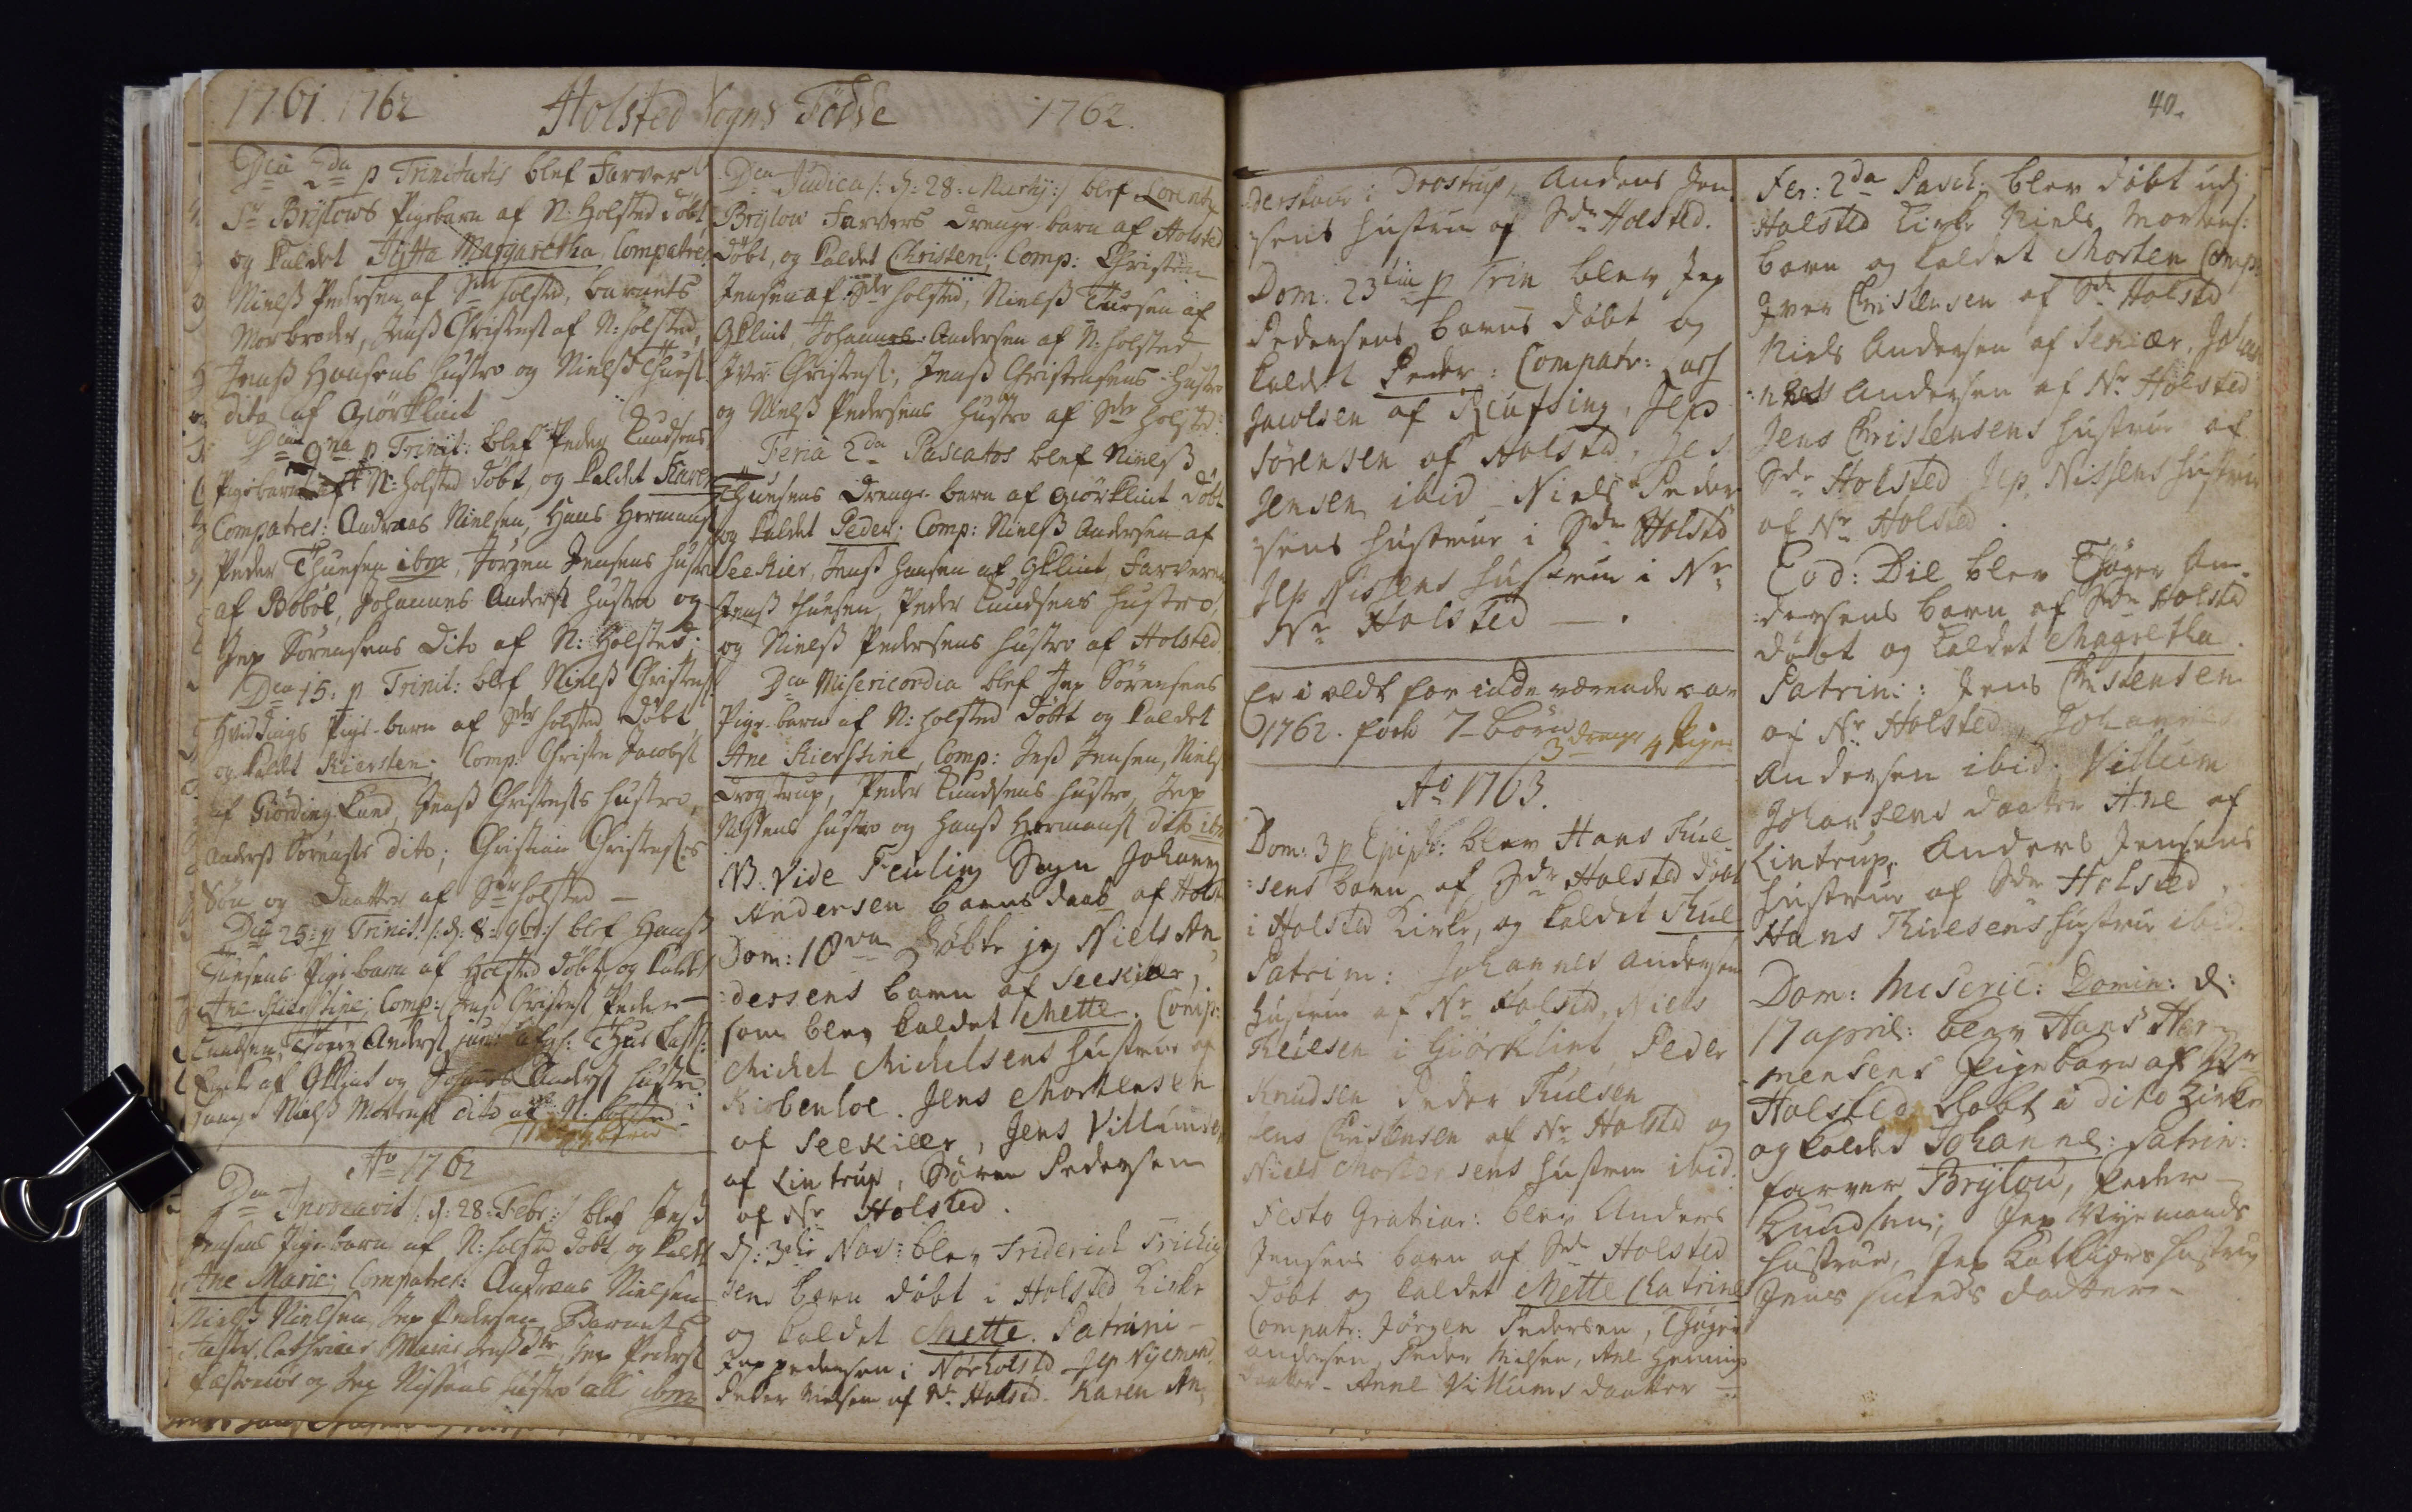1761. 1762
Holsted Sogns Fødde
Dca 2da p Trinitatis bleff Farver
Sr Brylows Pigebarn af N: Holsted døbt,
og kaldet Jytte Margaretha, Compatres
Nielss Pedersen af Sdr Holsted, barnets
Morbroder, Jenss Christens af N: Holsted,
Jenss Hansens Hustro og Nielss Thues
dito af Giørklint
Dca 9na p Trinit: blef Peder Knudsens
Pigebarn af N: Holsted døbt, og kaldet Karen
Compatres: Andræas Nielsen, Hans Hermans
Peder Thuesen ibm, Jørgen Jensens Hustr
af Bobøl, Johannes Anders Hustro og
Jep Sørensens Dito af N: Holsted.
Dca 15: p Trinit: blef Nielss Christens
Hviddings Pige-barn af Sdr Holsted døbt,
og kaldet Kiersten Comp: Christen Jacobs
af Giørding Lund, Jenss Christenss Hustro
Anderss, Sørenss dito; Christian Christenss
Søn og Daatter af Sdr Holsted -
Dca 25: p Trinit: /: d: 8= 9br: blef Hanss
Thuesens Pige barn af Holsted døbt, og kaldet
Ane Kierstine; Comp: Jess Christens, Peder
Knudsen Thoue Anders jun: Thue Lass:
Enke af Gklint og Johannes Anders Hustro
sampt Nielss Mortens af N: Holsted
## b##
Ao 1762
Dca Invocavit: d. 28. Febr: blef Jenss
Jensens Pige-barn af N: HolsteD dobt, og kaldet
Ane Marie; Compatres: Andræas Nielsen
Nielss Nielsen, Jep Pedersen Barnets
Faster. Cathrine Marie Jenssdtr Jep Peders
Fæstemøe og Jep Nissens Hustro alle ibm
1762.
Dom Judica /: d: 28: Martij :/ blef Lorentz
Brylou Farvers Drenge-barn af Holsted
døbt, og kaldet Christen; Comp: Christen
Jensen af Sdr Holsted, Niels Tuesen af
Gklint, Johannes Andersen af N: Holsted
Iver Christens, Jenss Christensens Hustro
og Nielss Pedersens Hustro af Sdr Holsted
Feria 2da Pascatos blef Nielss
Thuesens Drenge-barn af Giørklint døbt
og kaldet Peder; Comp: Nielss Andersen af
Seekier, Jenss Hansen af Gklint, Farveren
Jenss Thuesen, Peder Knudsens Hustro
og Nielss Pedersens Hustro af Holsted.
Dca Misericordia blef Jep Sørensens
Pige-barn af N: Holsted døbt og kaldet
Ane Kierstine, Comp: Jess Jensen, Niels
Drogstrup, Peder Knudsens Hustro, Jep
Nissens Hustro og Hanss Hermans dito ibm
NB: Vide Feuling Sogn Johanne
Andersen Barns daab af Holst
Dom: 18va Døbte jeg Niels An¬
dersens barn af Seekier,
som blev kaldet Mette. Comp:
Michel Michelsens Hustrue af
Kiobenloe. Jens Mortensen
af Seekieer, Jens Villumsen
af Lintrup, Søren Pedersen
af Nr Holsted.
d: 3die Nov: blev Friderich Fri##
sens barn døbt i Holsted Kirke
og kaldet Mette. Patrini
Jep Pedersen i Nørholsted Jep Nyeman
Peder Nielsen af Nr Holsted. Karen An¬
ders ## i Drostrup, Anders Jen¬
sens Hustru af Sdr Holdsted.
Dom: 23tia p Trin blev Jep
Pedersens barn døbt og
kaldet Peder: Compatr: Lars
Jacobsen af Reufsing, Jep
Sørensen af Holsted, Jes
Jensen ibid  Niels Peders¬
sens Hustrue i Sdr Holsted
Jep Nissens Hustrue i Nr
Nr Holsted
Er i aldt for inde værende aar
1762. fode 7 Børn
3 Drenge 4 Piger
Ao 1763.
Dom: 3 p Epiph: blev Hans Thue
= sens Barn af Sdr Holsted døbt
i Holsted Kirke, og kaldet Thue
Patrini: Johannes Andersens
Hustrue af Nr Holsted, Niels
Thuesen i Giørklint Peder
Knudsen Peder Thuesen
Jens Christensen af Nr Holsted og
Niels Mortensens Hustrue ibid:
Festo Gratiar: blev Anders
Jensens Barn af Sdr Holsted
døbt og kaldet Mette Chatrine
Compatr: Jørgen Pedersen, Thyge
Andersen Peder Nielssen, Ane Hennings
Daatter - Anne Villums Daatter
40
Fer: 2da Pasch: blev døbt udi
Holsted Kirke Niels Mortens:
Barn og kaldet Morten Comp:
Iver Christensen af Sdr Holsted
Niels Andersen af Sekiær, Johan
= nes Andersen af Nr Holsted
Jens Christensens Hustrue af
Sdr Holsted Jep Nielsens Hustrue
af Nr Holsted
Eod: Die blev Thøger An¬
= dersens Barn af Sdr Holsted
døbt og kaldet Magretha
Patrini: Jens Christensen
af Nr Holsted: Johannes
Andersen ibid. Villum
Johansens Daatter Ane af
Lintrup Anders Jensens
Hustrue af Sdr Holsted
Hans Thuesens Hustrue ibid.
Dom: Miseric: Domin: d:
17 April: blev Hans Hen¬
mensens Pige Barn af Sdr
Holsted døbt i Dito Kirke
og kaldet Johanne: Patrin:
farver Brylou, Peder
Knudsen, Jep Nyemands
Hustrue, Jep Katkiærs Hustrue
Jens Smeds datter
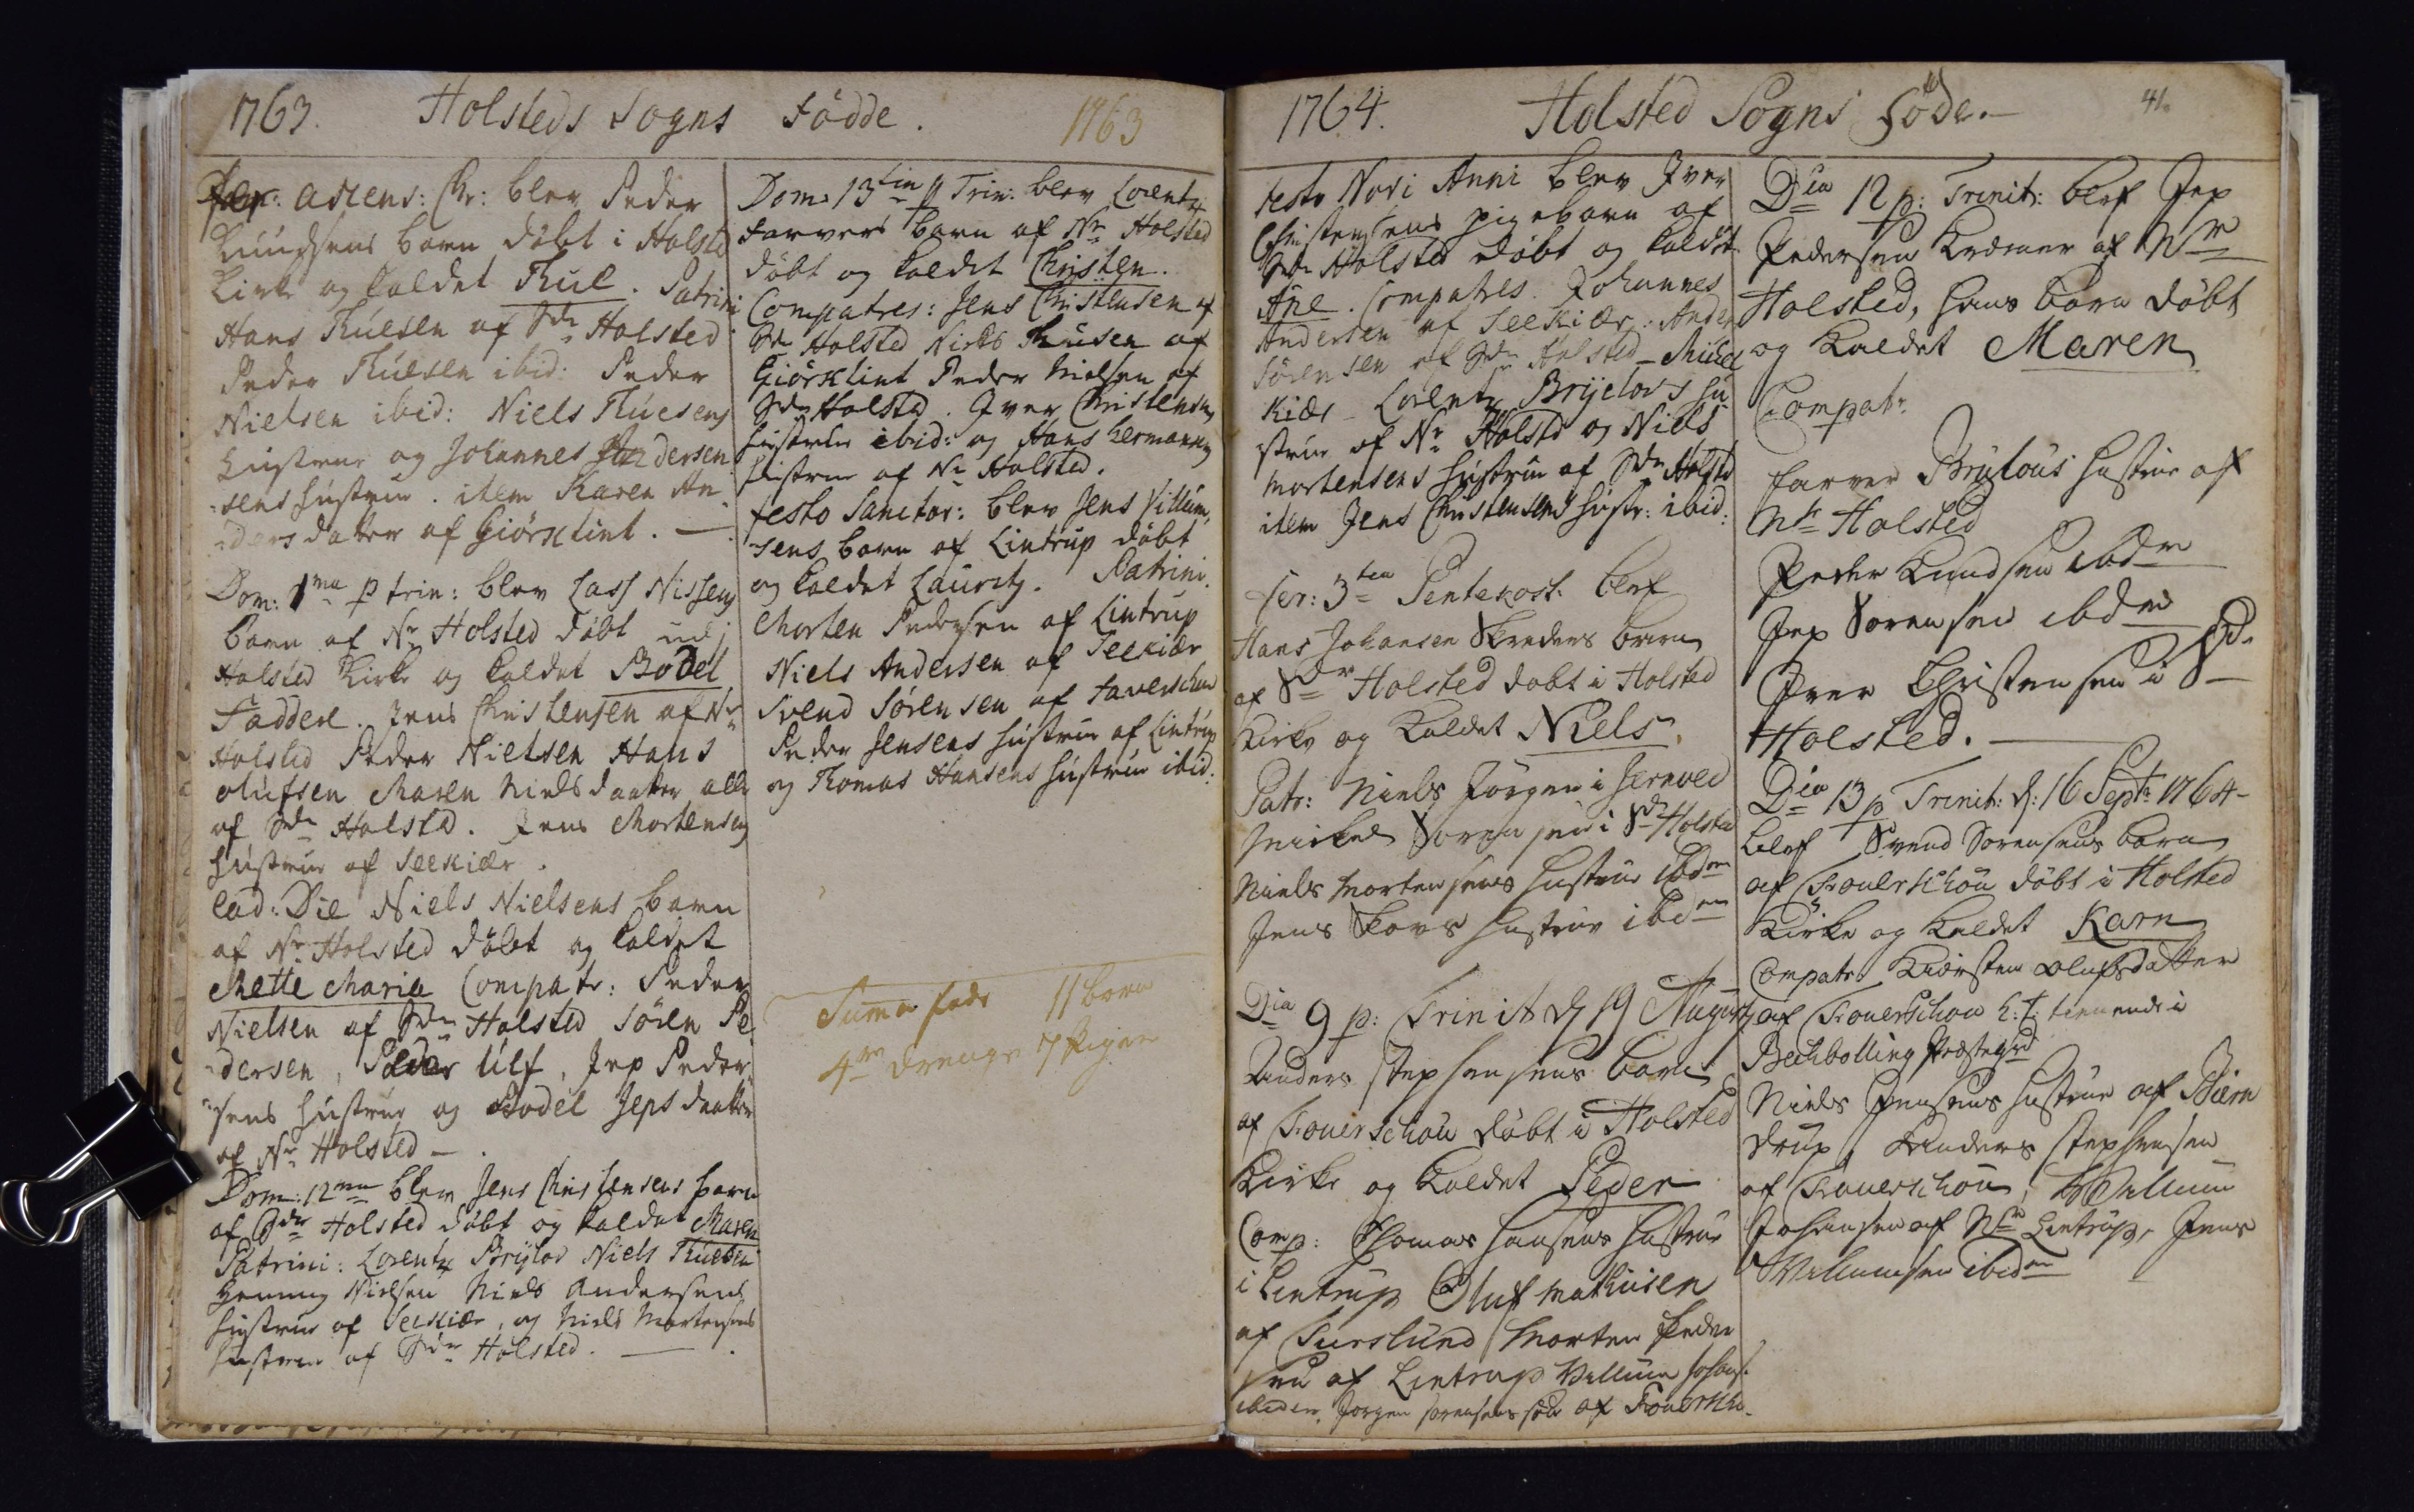Holsted Sogns Fødde.
1763.
Fer: Ascens: Chr: blev Peder
Knudsens barn døbt i Holsted
Kirke og kaldet Thue. Patrini
Hans Thuesen af Sdr Holsted
Peder Thuesen ibid: Peder
Nielsen ibid: Niels Thusens
Hustrue og Johannes Andersen
sens Hustrue. item Karen An¬
dersdatter af Giørklint.
Dom: 1ma p trin: blev Lass Nissens
Barn af Nr Holsted døbt udi
Holsted Kirke og kaldet Bodel
Faddere.  Jens Christensen af Nr
Holsted Peder Nielsen Hans
Olufsen Maren Nielsdaatter alle
af Sdr Holsted. Jens Mortensens
Hustrue af Seekiær
Ead: Die Niels Nielsens barn
af Nr Holsted døbt og kaldet
Mette Maria Compatr: Peder
Nielsen af Sdr Holsted Søren Pe¬
dersen, Peder Ulf, Jep Peder¬
sens Hustrue og Bodel Jepsdatter
af Nr Holsted -
Dom: 12ma blev Jens Christensens barn
af Sdr Holsted døbt og kaldet Maren
Patrini: Lorentz Brylov Niels Thusen
Henning Nielsen Niels Andersens
Hustrue af Seekiær, og Niels Mortensens
Hustrue af Sdr Holsted.
1763
Dom: 13tie p Trin: blev Lorentz
farvers barn af Nr Holsted
døbt og kaldet Christen.
Compatres: Jens Christensens af
Sdr Holsted Niels Thuesen af
Giørklint Peder Nielsen af
Sdr Holsted. Iver Christensens
Hustrue ibid: og Hans Hermansen
Hustrue af Nr Holsted.
Festo Sanctor: blev Jens Villum¬
sens Barn af Lintrup døbt
og kaldet Lauritz. Patrine.
Morten Pedersen af Lintrup
Niels Andersen af Seekiær
Svend Sørensen af Faverschov
Peder Jensens Hustrue af Lintrup
og Thomas Hansens Hustrue ibid:
Summa fode 11 Born
4## Drenge 7 Piger
Holsted Sogns Føde
1764
Festo Novi Anni blev Iver
Christensens pigebarn af
Sdr Holsted døbt og kaldet
Ane Compatres: Johannes
Andersen af Seekiær: Anders
Sørensen af Sdr Holsted _ ##¬
Kiær - Lorentz Bryelovs Hu¬
strue af Nr Holsted og Niels
Mortensens Hustrue af Sdr Holsted
item Jens Christensens Hustr: ibid
Fer: 3tia Pentekost: blef
Hans Johansen Skreders barn
af Sdr Holsted døbt i Holsted
Kirke og kaldet Niels.
Patr: Niels Jørgen i Jernved
Mickel Sorensen i Sdr Holsted
Niels Mortensens Hustrue ibdm
Jens Skovs Hustrue ibdm
Dca 9 p: Trinit d 19 Augustj
Anders Stephensens barn
af Fouerschou døbt i Holsted
Kirke og kaldet Peder
Comp: Thomas Hansens Hustrue
i Lintrup Oluf Matthiisen
af Tiirslund Morten Peder
sen af Lintrup Villum Johans
ibidem Jorgen Sorensens Søn af Fouer##
Dca 12 p: Trinit: blef Jep
Pedersen Kræmer af Nr
Holsted, hans barn døbt
og kaldet Maren
Compat:
farver Brulous Hustrue af
Nr Holsted
Peder Knudsen ibdm
Jep Sorensen ibdm
Iver Christensen i Sdr
Holsted.
Dca 13 p Trinit: d: 16 Septr 1764 -
blef Svend Sorensens barn
af FouerschouProulsøn døbt i Holsted
Kirke og kaldet Karn
Compatr Kiærsten Olufsdatter
af Fouerschou ##: t: tienende i
Bechbolling Præstegrd
Niels Jensens Hustrue af Pl##
strup, Anders Stephensen
af Fouerschou Villum
Johansen af Nr Lintrup, Jens
Villumsen ibidm